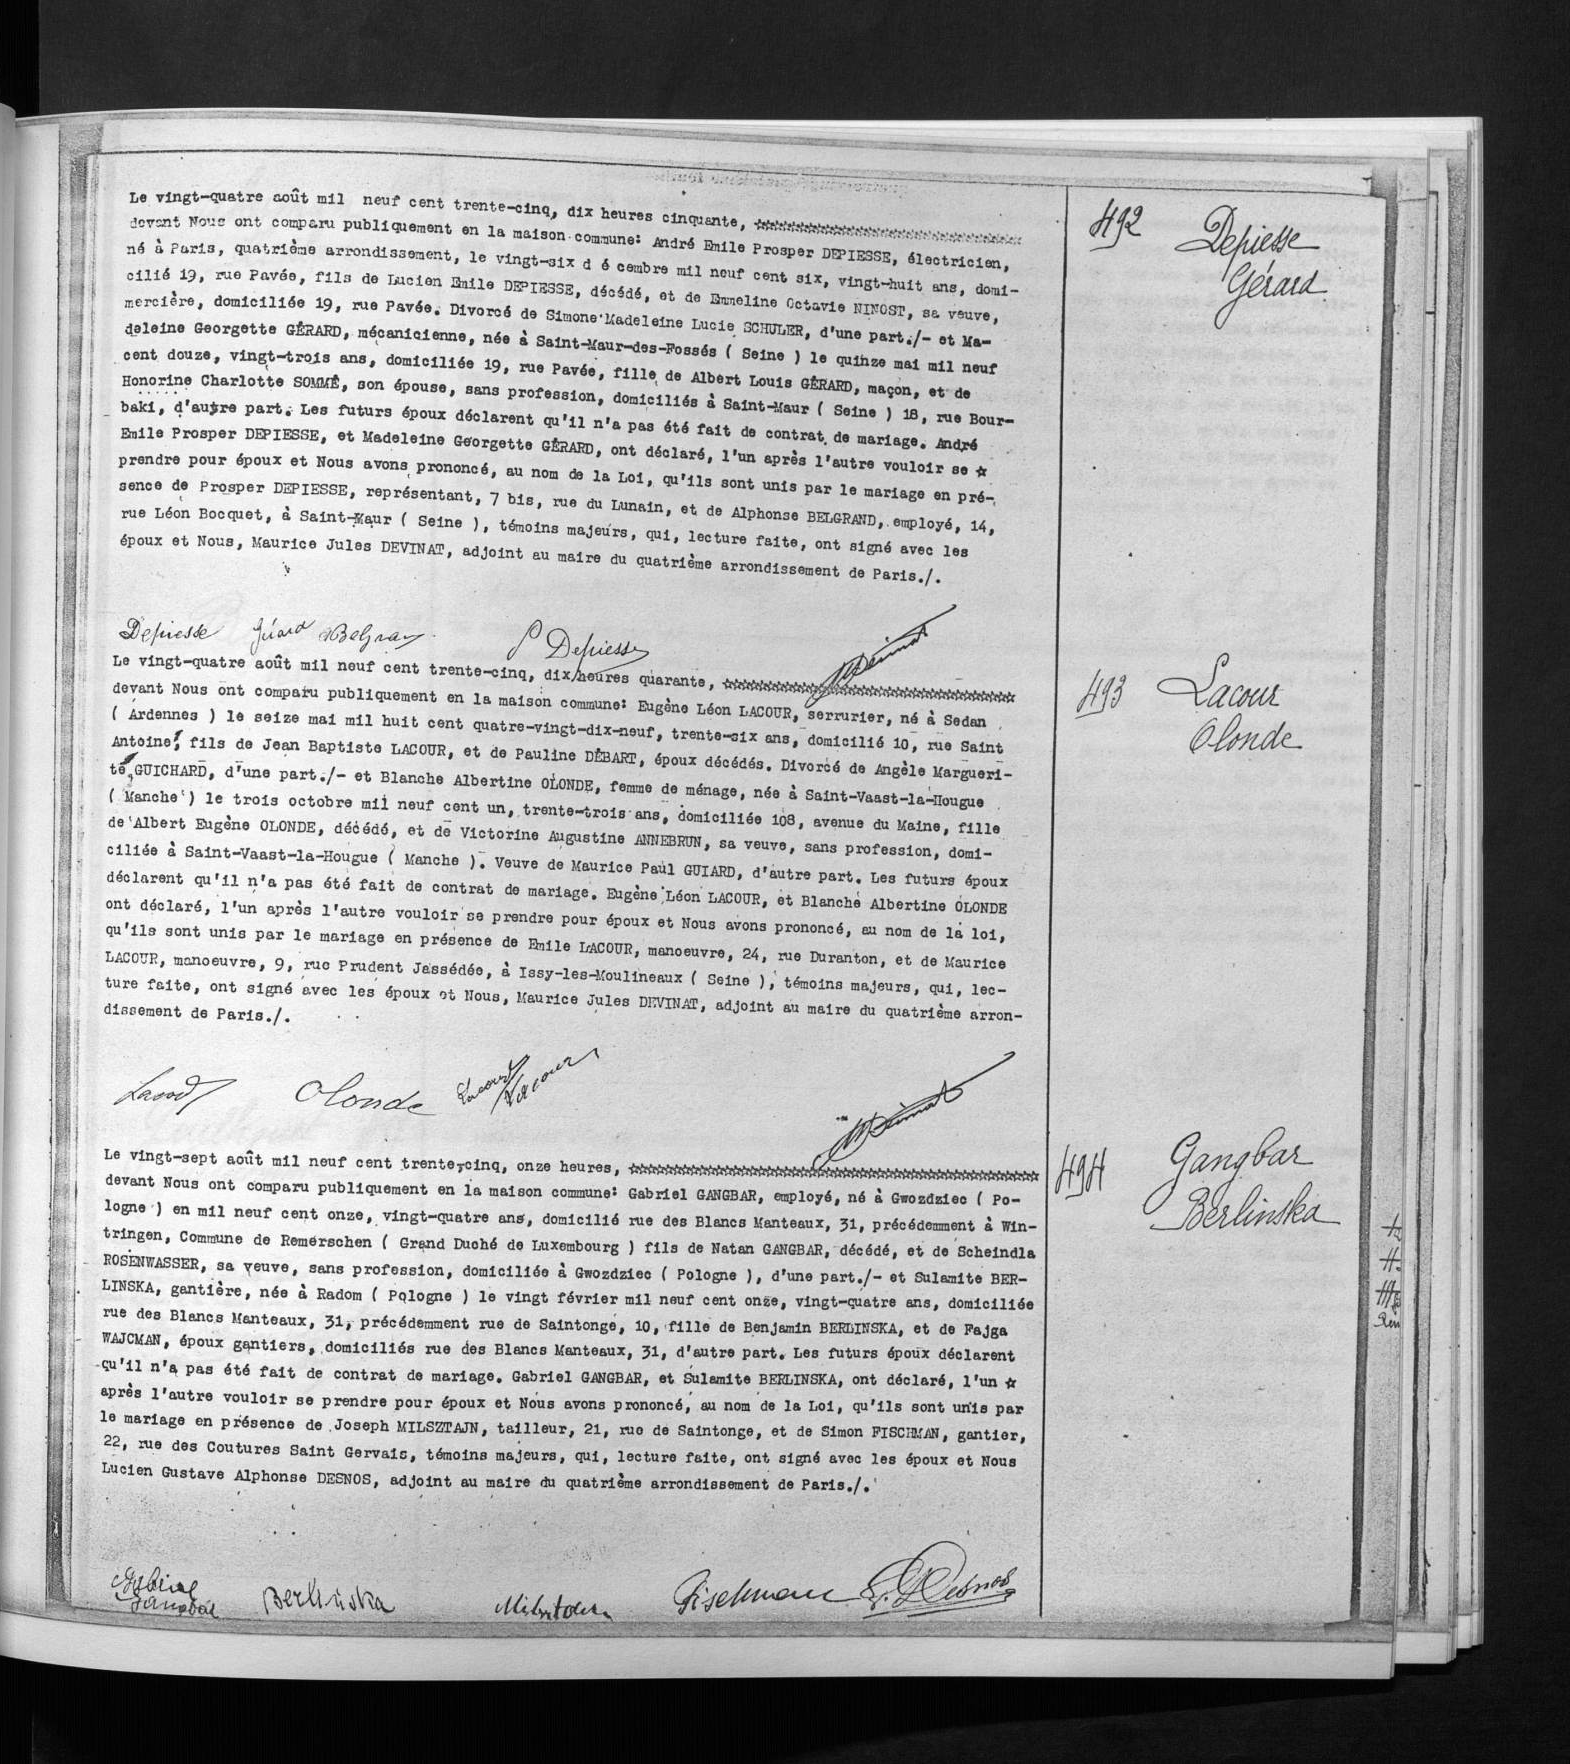Le vingt-quatre août mil neuf cent trente-cinq, dix heures cinquante, ****
devant Nous ont comparu publiquement en la maison commune: André Emile Prosper DEPIESSE, électricien,
né à Paris, quatrième arrondissement, le vingt-six d é cembre mil neuf cent six, vingt-huit ans, domi-
cilié 19, rue Pavée, fils de Lucie Emile DEPIESSE, décédé, et de Emmeline Octavie NINOST, sa veuve,
mercière, domicilié 19, rue Pavée. Divorcé de Simone Madeleine Lucie SCHULER, d'une part ./-et Ma-
deleine Georgette GERARD, mécanicienne, née à Saint-Maur-des-Fossés (Seine) le quinze mai mil neuf
cent douze, vingt-trois ans, domiciliée 19, rue Pavée, fille de Albert Louis GERARD, maçon, et de
Honorine Charlotte SOMME, son épouse, sans profession, domiciliés à Saint-Maur (Seine) 18, rue Bour-
baki, d'autre part. Les futurs époux déclarent qu'il n'apas été fait de contrat de mariage. André
Emile Prosper DEPIESSE, et Madeleine Georgette GERARD, ont déclaré, l'un après l'autre vouloir se *
prendre pour époux et Nous avons prononcé, au nom de la Loi, qu'ils sont unis par le mariage en pré-
sence de Prosper DEPIESSE, représentant, 7 bis, rue du Lunain, et de Alphone BELGRAND, employé, 14,
rue Léon Bocquet, à Saint-Kaur (Seine), témoins majeurs, qui, lecture faite, ont signé avec les
époux et Nous, Maurice Jules DEVINAT, adjoint au maire du quatrième arrondissement de Paris ./.
Le vingt-quatre août mil neuf cent trente-cinq, dix heures quarante, ****
devant Nous ont comparu publiquement en la maison commune: Eugène Léon LACOUR, serrurier, né à Sedan
(Ardennes) le seize mai mil huit cent quatre-vingt-dix-neuf, trente-six ans, domicilié 10, rue Saint
Antoine, fils de Jean Baptiste LACOUR, et de Pauline DEBART, époux décédés. Divorcé de Angèle Margueri-
te GUICHARD, d'une part ./-et Blanche Albertine OLONDE, femme de ménage, néa à Saint-Vaast-la-IIougue
(Manche) le trois octobre mil neuf cent un, trente-trois ans, domiciliée 108, avenue du Maine, fille
de Albert Eugène OLONDE, décédé, et de Victorine Augustine ANNEBRUN, sa veuve, sans profession, domi-
ciliée à Saint-Vaast-la-Hougue (Manche). Veuve de Maurice Paul GUIARD, d'autre part. Les futurs époux
déclarent qu'il n'a pas été fait de contrat de mariage. Eugène Léon LACOUR, et Blanche Albertine OLONDE
ont déclaré, l'un après l'autre vouloir se prendre pour époux et Nous avons pronnoncé, au nom de la loi,
qu'ils sont unis par le mariage en présence de Emile LACOUR, manoeuvre, 24, rue Duranton, et de Maurice
LACOUR, manoeuvre, 9, rue prudent Jassédée, à Issy-les-Moulineaux (Seine), témoins majeurs, qui, lec-
ture faite, ont signé avec les époux et Nous, Maurice Jules DEVINAT, adjoint au maire du quatrième arron-
dissement de Paris ./.
Le vingt-sept août mil neuf cent trente-cinq, onze heures, ****
devant Nous ont comparu publiquement en la maison commune: Gabriel GANGBAR, employé, né à Gwozdziec (Po-
logne) en mil neuf cent onze, vingt-quatre ans, domicilié rue des Blancs Manteaux, 31, précédemment à Win-
tringen, Commune de Remerschen (Grand Duché de Luxembourg) fils de Natan GANGBAR, décédé, et de Scheindla
ROSENWASSER, sa veuve, sans profession, domiciliée à Gwozdziec (Pologne), d'une part ./-et Sulamite BER-
LINSKA, gantière, née à Radom (Pologne) le vingt février mil neuf cent onze, vingt-quatre ans, domiciliée
rue des Blancs Manteaux, 31, précédemment rue de Saintonge, 10, fille de Benjamin BERDINSKA, et de Fajga
WAJCAM, époux gantiers, domiciliés rue des Blancs Manteaux, 31, d'autre part. Les futurs époux déclarent
qu'il n'a pas été fait de contrat de mariage. Gabriel GANGBAR, et Sulamite BERLINSKA, ont déclaré, l'un*
après l'autre vouloir se prendre pour époux et Nous avons prononcé, au nom de la Loi, qu'ils sont unis par
le mariage en présence de Joseph MILSZTAN, tailleur, 21, rue de Saintonge, et de Simon FISCHMAN, gantier,
22, rue des Coutures Saint Gervais, témoins majeurs, qui, lecture faite, ont signé avec les époux et Nous
Lucien Gustave Alphone DESNOS, adjoint au maire du quatrième arrondissement de Paris ./.